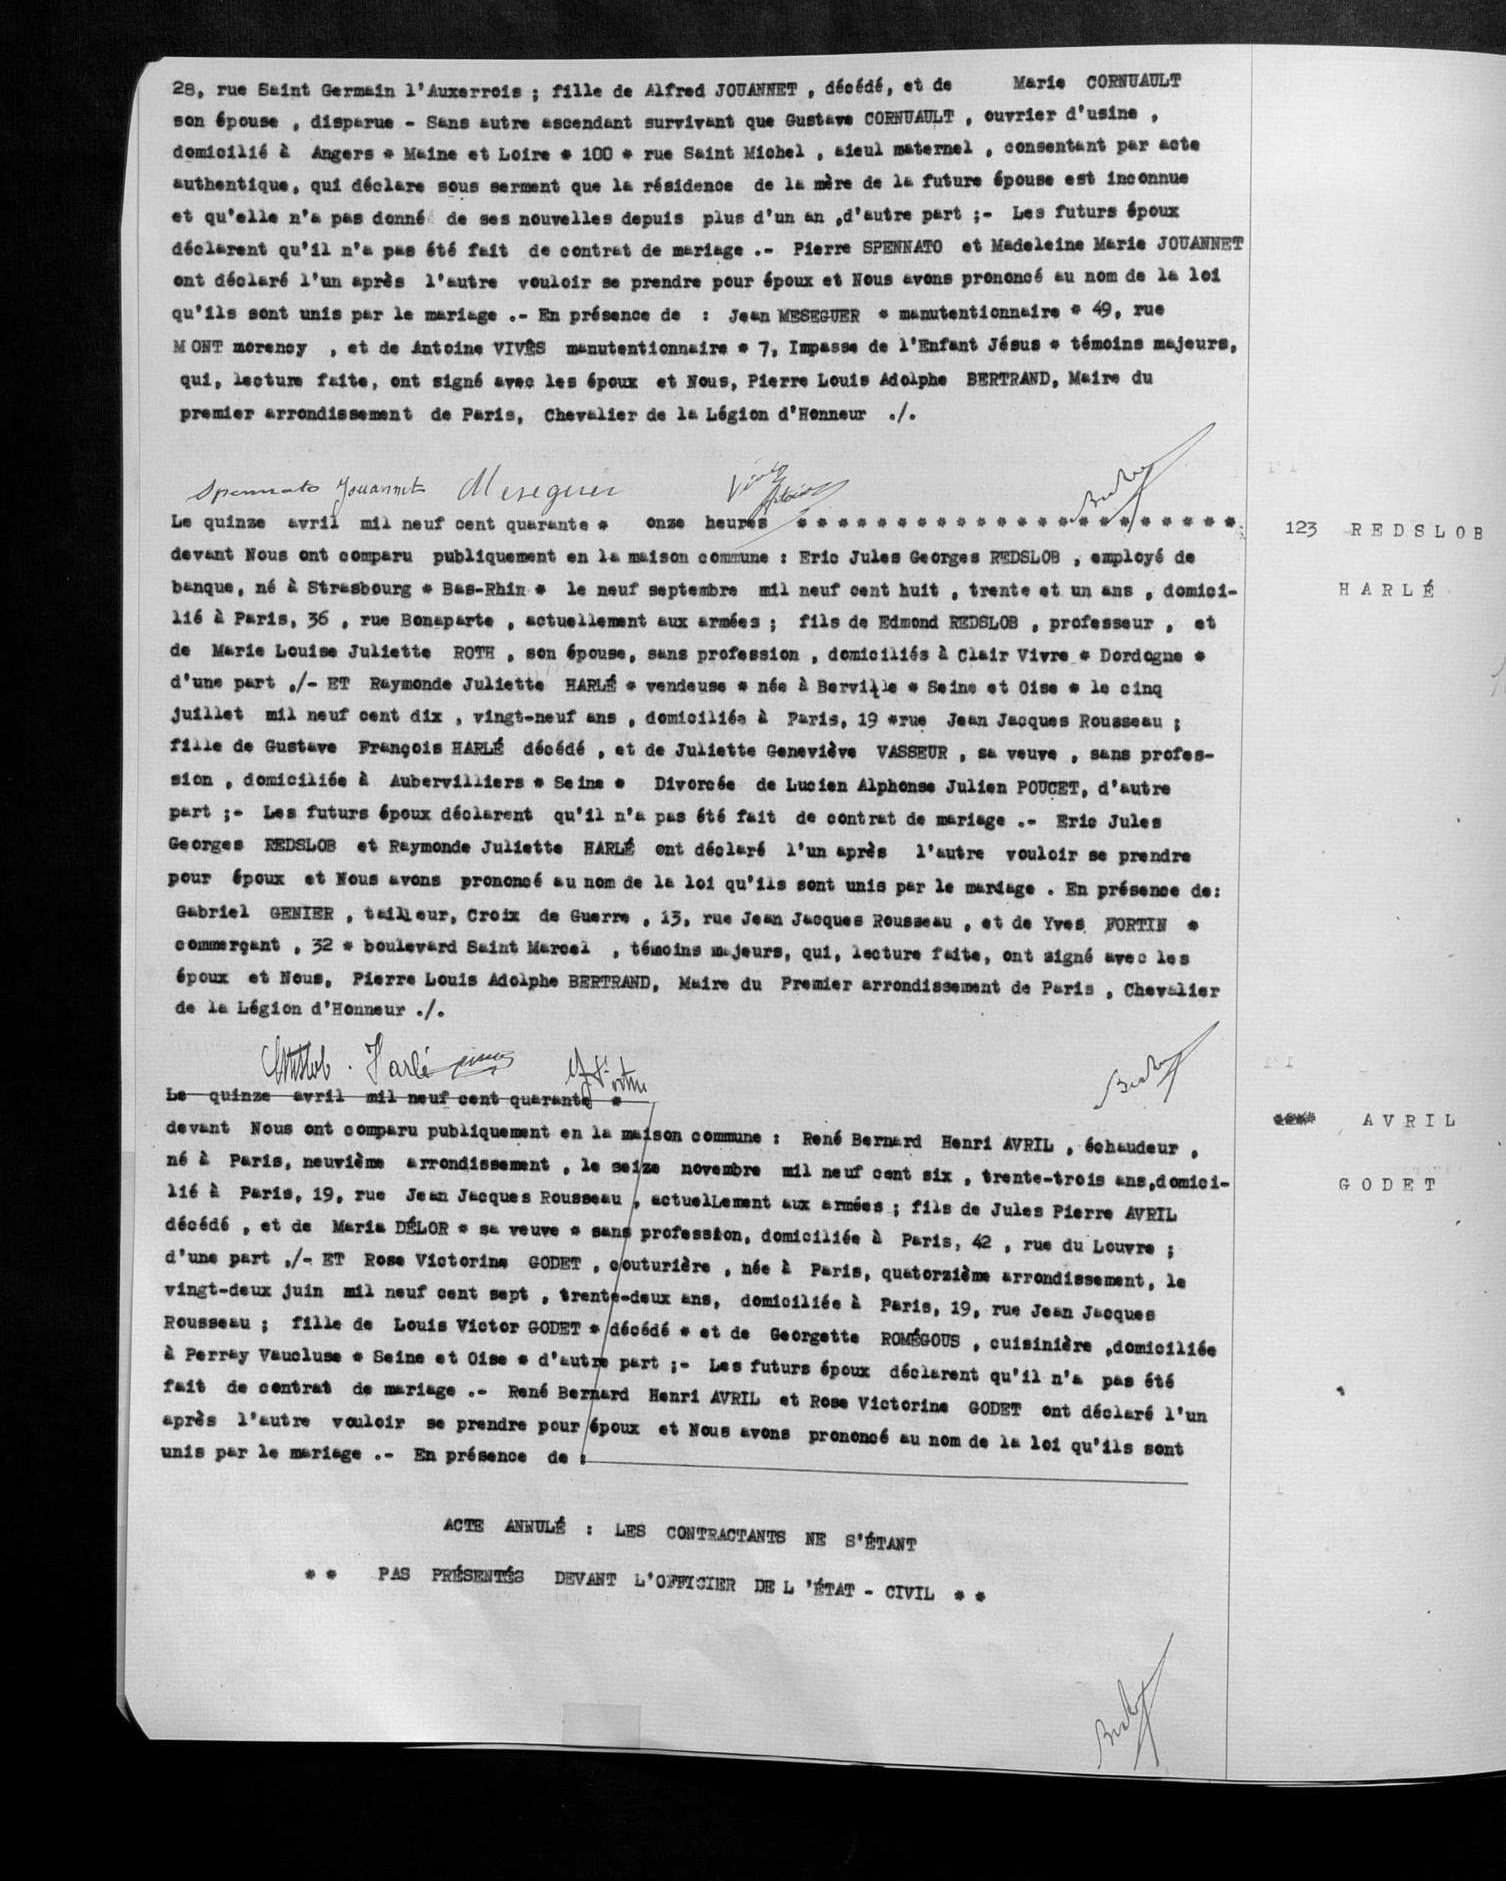28, rue Saint Germain l'Auxerrois; fille de Alfred JOUANNET, décédé, et de Marie CORNUAULT
son épouse, disparu-Sans autre ascendant survivant que Gustave CORNUAULT, ouvrier d'usine,
domicilié à Angers * Maine et Loire * 100 * rue Saint Michel, aieul maternel, consentant par acte
authentique, qui déclare sous serment que la résidence de la mère de la future épouse est inconnue
et qu'elle n'a pas donné de ses nouvelles depuis plus d'un an, d'autre part ;-Les futurs époux
déclarent qu'il n'a pas été fait de contrat de mariage .-Pierre SPENNATO et Madeleine Marie JOUANNET
ont déclaré l'un après l'autre vouloir se prendre pour époux et Nous avons prononcé au nom de la loi
qu'ils sont unis par le mariage .-En présence de: Jean MESEGUER * manutentionnaire * 49, rue
MONT morenoy, et de Antoine VIVES manutentionnaire * 7, Impasse de l'Enfant Jésus * témoins majeurs,
qui, lecture faite, ont signé avec les époux et Nous, Pierre Louis Adolphe BERTRAND, Maire du
premier arrondissement de Paris, Chevalier de la Légion d'Honneur ./.
Le quinze avril mil neuf cent quarante * onze heures ****
devant Nous ont comparu publiquement en la maison commune: Eric Jules Georges REDSLOB, employé
de banque, né à Strasnourg * Bas-Rhin * le neuf septembre mil neuf cent huit, trente et un ans, domici-
lié à Paris, 36, rue Bonaparte, actuellement aux armées; fils de Edmond REDSLOB, professeur, et
de Marie Louise Juliette ROTH, son épouse, sans profession, domiciliés à Clair Vivre * Dordogne *
d'une part ,/-ET Raymonde Juliette HARLE * vendeuse * née à Berville * Seine et Oise * le cinq
juillet mil neuf cent dix, vingt-neuf ans, domiciliée à Paris, 19 * rue Jean Jacques Rousseau;
fille de Gustave François HARLE décédé, et de Juliette Geneviève VASSEUR, sa veuve, sans profes-
sion, domiciliée à Aubervilliers * Seine * Divorcée de Lucien Alphonse Julien POUCET, d'autre
part;-Les futurs époux déclarent qu'il n'a pas été fait de contrat de mariage .-Eric Jules
Georges REDSLOB et Raymonde Juliette HARLE ont déclaré l'un après l'autre vouloir se prendre
pour époux et Nous avons prononcé au nom de la loi qu'ils sont unis par le mariage. En présence de:
Gabriel GENIER, tailleur, Croix de Guerre, 13, rue Jean Jacques Rousseau, et de Yves FORTIN *
commerçant, 32 * boulevard Saint Marcel, témoins majeurs, qui, lecture faite, ont signé avec les
époux et Nous, Pierre Louis Adolphe BERTRAND, Maire du Premier arrondissement de Paris, Chevalier
de la Légion d'Honneur ./.
Le quinze avril mil neuf cent quarante *
devant Nous ont comparu publiquement en la maison commune: René Bernard Henri AVRIL, échaudeur,
né à Paris, neuvième arrondissement, le seize novembre mil neuf cent six, trente-trois ans, domici-
lié à Paris, 19, rue Jean Jacques Rousseau, actuellement aux armées; fils de Jules Pierre AVRIL
décédé, et de Maria DELOR * sa veuve * sans profession, domiciliée à Paris, 42, rue du Louvre;
d'une part ,/-ET Rose Victorine GODET, couturière, née à Paris, quatorzième arrondissement, le
vingt-deux juin mil neuf cent sept, trente-deux ans, domiciliée à Paris, 19, rue Jean Jacques
Rousseau; fille de Louis Victor GODET * décédé * et de Georgette ROMEGOUS, cuisinière, domiciliée
à Perray Vaucluse * Seine et Oise * d'autre part ;-Les futurs époux déclarent qu'il n'a pas été
fait de contrat de mariage .-René Bernard Henri AVRIL et Rose Victorine GODET ont déclaré l'un
après l'autre vouloir se prendre pour époux et Nous avons prononcé au nom de la loi qu'ils sont
unis par le mariage .-En présence de:
ACTE ANNULE: LES CONTRACTANTS NE S'ETANT
** PAS PRESENTES DEVANT L'OFFICIER DE L'ETAT-CIVIL **
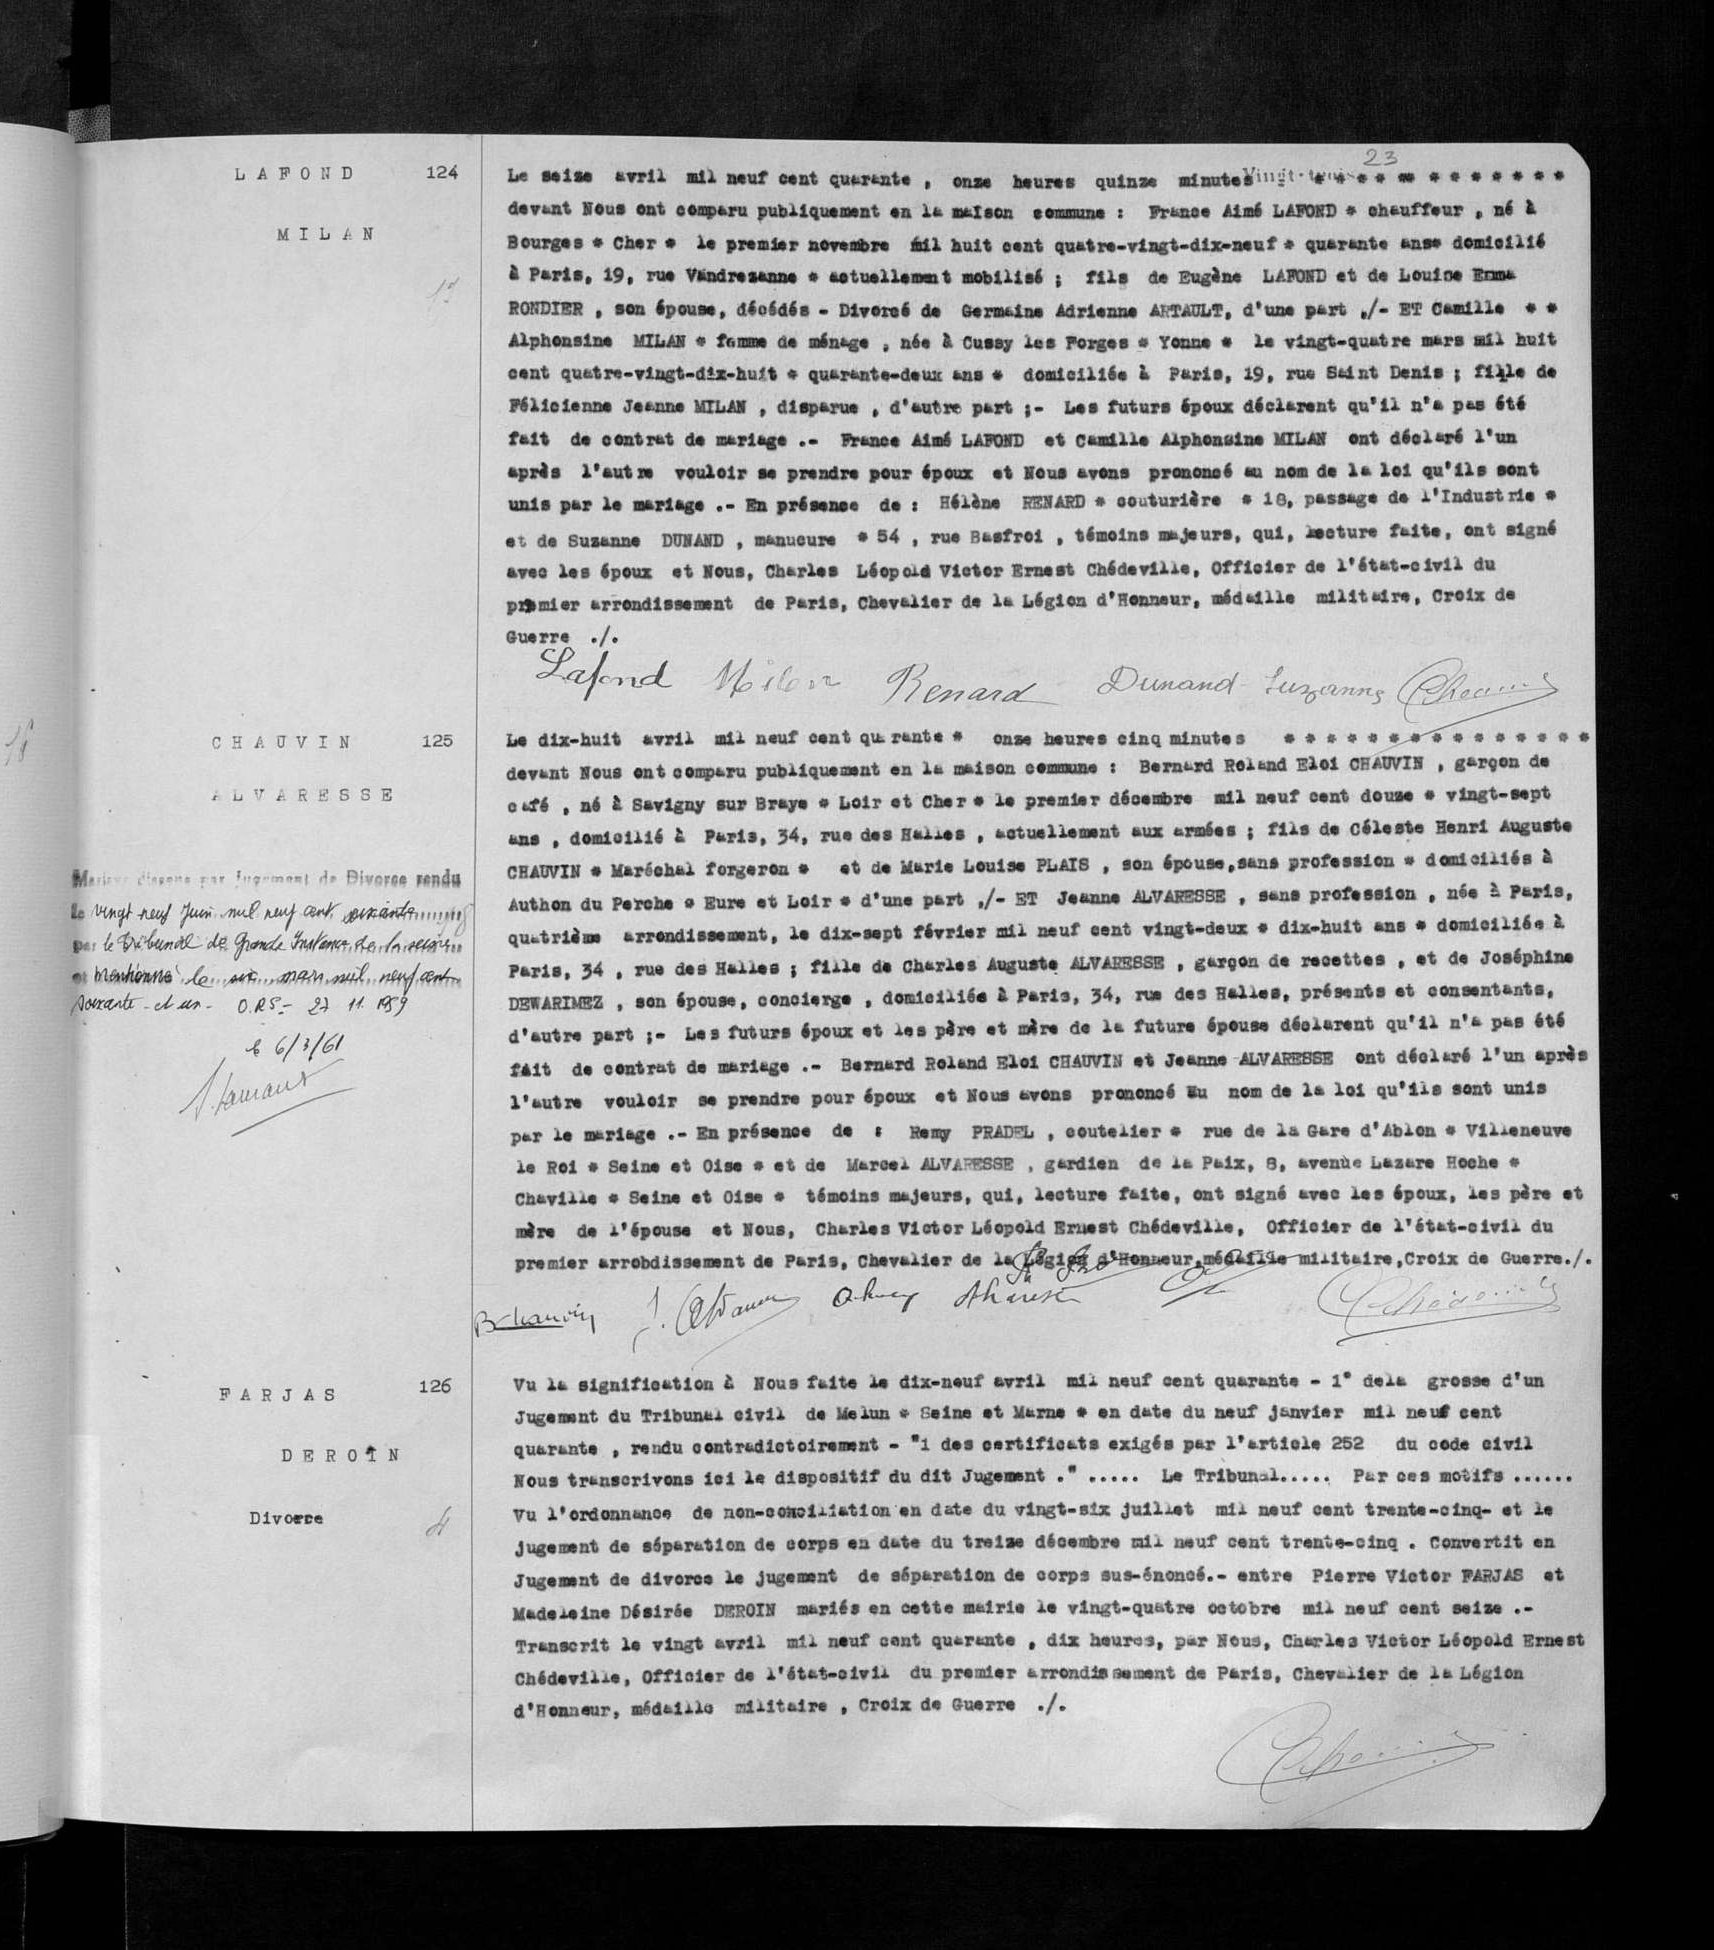Le seize avril mil neuf cent quarante, onze heures quinze minutes ****
devant Nous ont comparu publiquement en la maison commune: France Aimé LAFOND * chauffeur, né à
Bourges * Cher * le premier novembre mil huit cent quatre-vingt-dix-neuf * quarante ans * domicilié
à Paris, 19, rue Vandrezanne * actuellement mobilisé; fils de Eugène LAFOND et de Louise Emma
RONDIER, son épouse, décédés-Divorcé de Germaine Adrienne ARTAULT, d'une part ,/-ET Camille **
Alphonsine MILAN * femme de ménage, née à Cusay Les Forges * Yonne * le vingt-quatre mars mil huit
cent quatre-vingt-dix-huit * quarante-deux ans * domiciliée à Paris, 19, rue Saint Denis; fille de
Félicienne Jeanne MILAN, disparue, d'autre part ;-Les futurs époux déclarent qu'il n'a pas été
fait de contrat de mariage .-France Aimé LAFOND et Camille Alphonsine MILAN ont déclaré l'un
après l'autre vouloir se prendre pour époux et Nous avons prononcé au nom de la loi qu'ils sont
unis par le mariage .-En présence de: Hélène RENARD * couturière * 18, passage de l'Industrie *
et de Suzanne DUNAND, manucure * 54, rue Basfroi, témoins majeurs, qui, lecture faite, ont signé
avec les époux et Nous, Charles Léopold Victor Ernest Chédeville, Officier de l'état-civil du
premier arrondissement de Paris, Chevalier de la Légion d'Honneur, médaille militaire, Croix de
Guerre ./.
Le dix-huit avril mil neuf cent quarante * onze heures cinq minutes ****
devant Nous ont comparu publiquement en la maison commune: Bernard Roland Eloi CHAUVIN, garçon de
café, né à Savigny sur Braye * Loir et Cher * le premier décembre mil neuf cent douze * vingt-sept
ans, domicilié à Paris, 34, rue des Halles, actuellement aux armées; fils de Céleste Henri Auguste
CHAUVIN * Maréchal forgeron * et de Marie Louise PLAIS, son épouse, sans profession * domiciliés à
Authon du Perche * Eure et Loir * d'une part ,/-ET Jeanne ALVARESSE, sans profession, née à Paris,
quatrième arrondissement, le dix-sept février mil neuf cent vingt-deux * dix-huit ans * domiciliés à
Paris, 34, rue des Halles; fille de Charles Auguste ALVARESSE, garçon de recettes, et de Joséphine
DEWARIMEZ, son épouse, concierge, domiciliée à Paris, 34, rue des Halles, présents et consentants,
d'autre part ;-Les futurs époux et les père et mère de la future épouse déclarent qu'il n'a pas été
fait de contrat de mariage .-Bernard Roland Loi CHAUVIN et Jeanne ALVARESSE ont déclaré l'un après
l'autre vouloir se prendre pour époux et Nous avons prononcé au nom de la loi qu'ils sont unis
par le mariage .-En présence de: Remy PRADEL, coutelier * rue de la Gare d'Ablon * Villeneuve
le Roi * Seine et Oise * et de Marcel ALVARESSE, gardien de la Paix, 8, avenue Lazare Hoche *
Chaville * Seine et Oise * témoins majeurs, qui, lecture faite, ont signé avec les époux, les père et
mère de l'épouse et Nous, Charles Victor Léopold Ernest Chédeville, Officier de l'état-civil du
premier arrondissement de Paris, Chevalier de la Légion d'Honneur, médaille militaire, Croix de Guerre ./.
Vu la signification à Nous faite le dix-neuf avril mil neuf cent quarante-1° de la grosse d'un
Jugement du Tribunal civil de Melun * Seine et Marne * en date du neuf janvier mil neuf cent
quarante, rendu contradictoirement-" 'i des certificats exigés par l'article 252 du code civil
Nous transcrivons ici le dispositif du dit Jugement. ''..... Le Tribunal.... Par ces motifs.....
Vu l'ordonnance de non-conciliation en date du vingt-six juillet mil neuf cent trente-cinq-et le
jugement de séparation de corps en date du treize décembre mil neuf cent trente-cinq. Convertit en
Jugement de divorce le jugement de séparation de corps sus-énoncé .-entre Pierre Victor FARJAS et
Madeleine Désirée DEROIN mariés en cette mairie le vingt-quatre octobre mil neuf cent seize.
Transcrit le vingt avril mil neuf cent quarante, dix heures, par Nous, Charles Victor Léopold Ernest
Chédeville, Officier de l'état-civil du premier arrondissement de Paris, Chevalier de la Légion
d'Honneur, médaille militaire, Croix de Guerre ./.